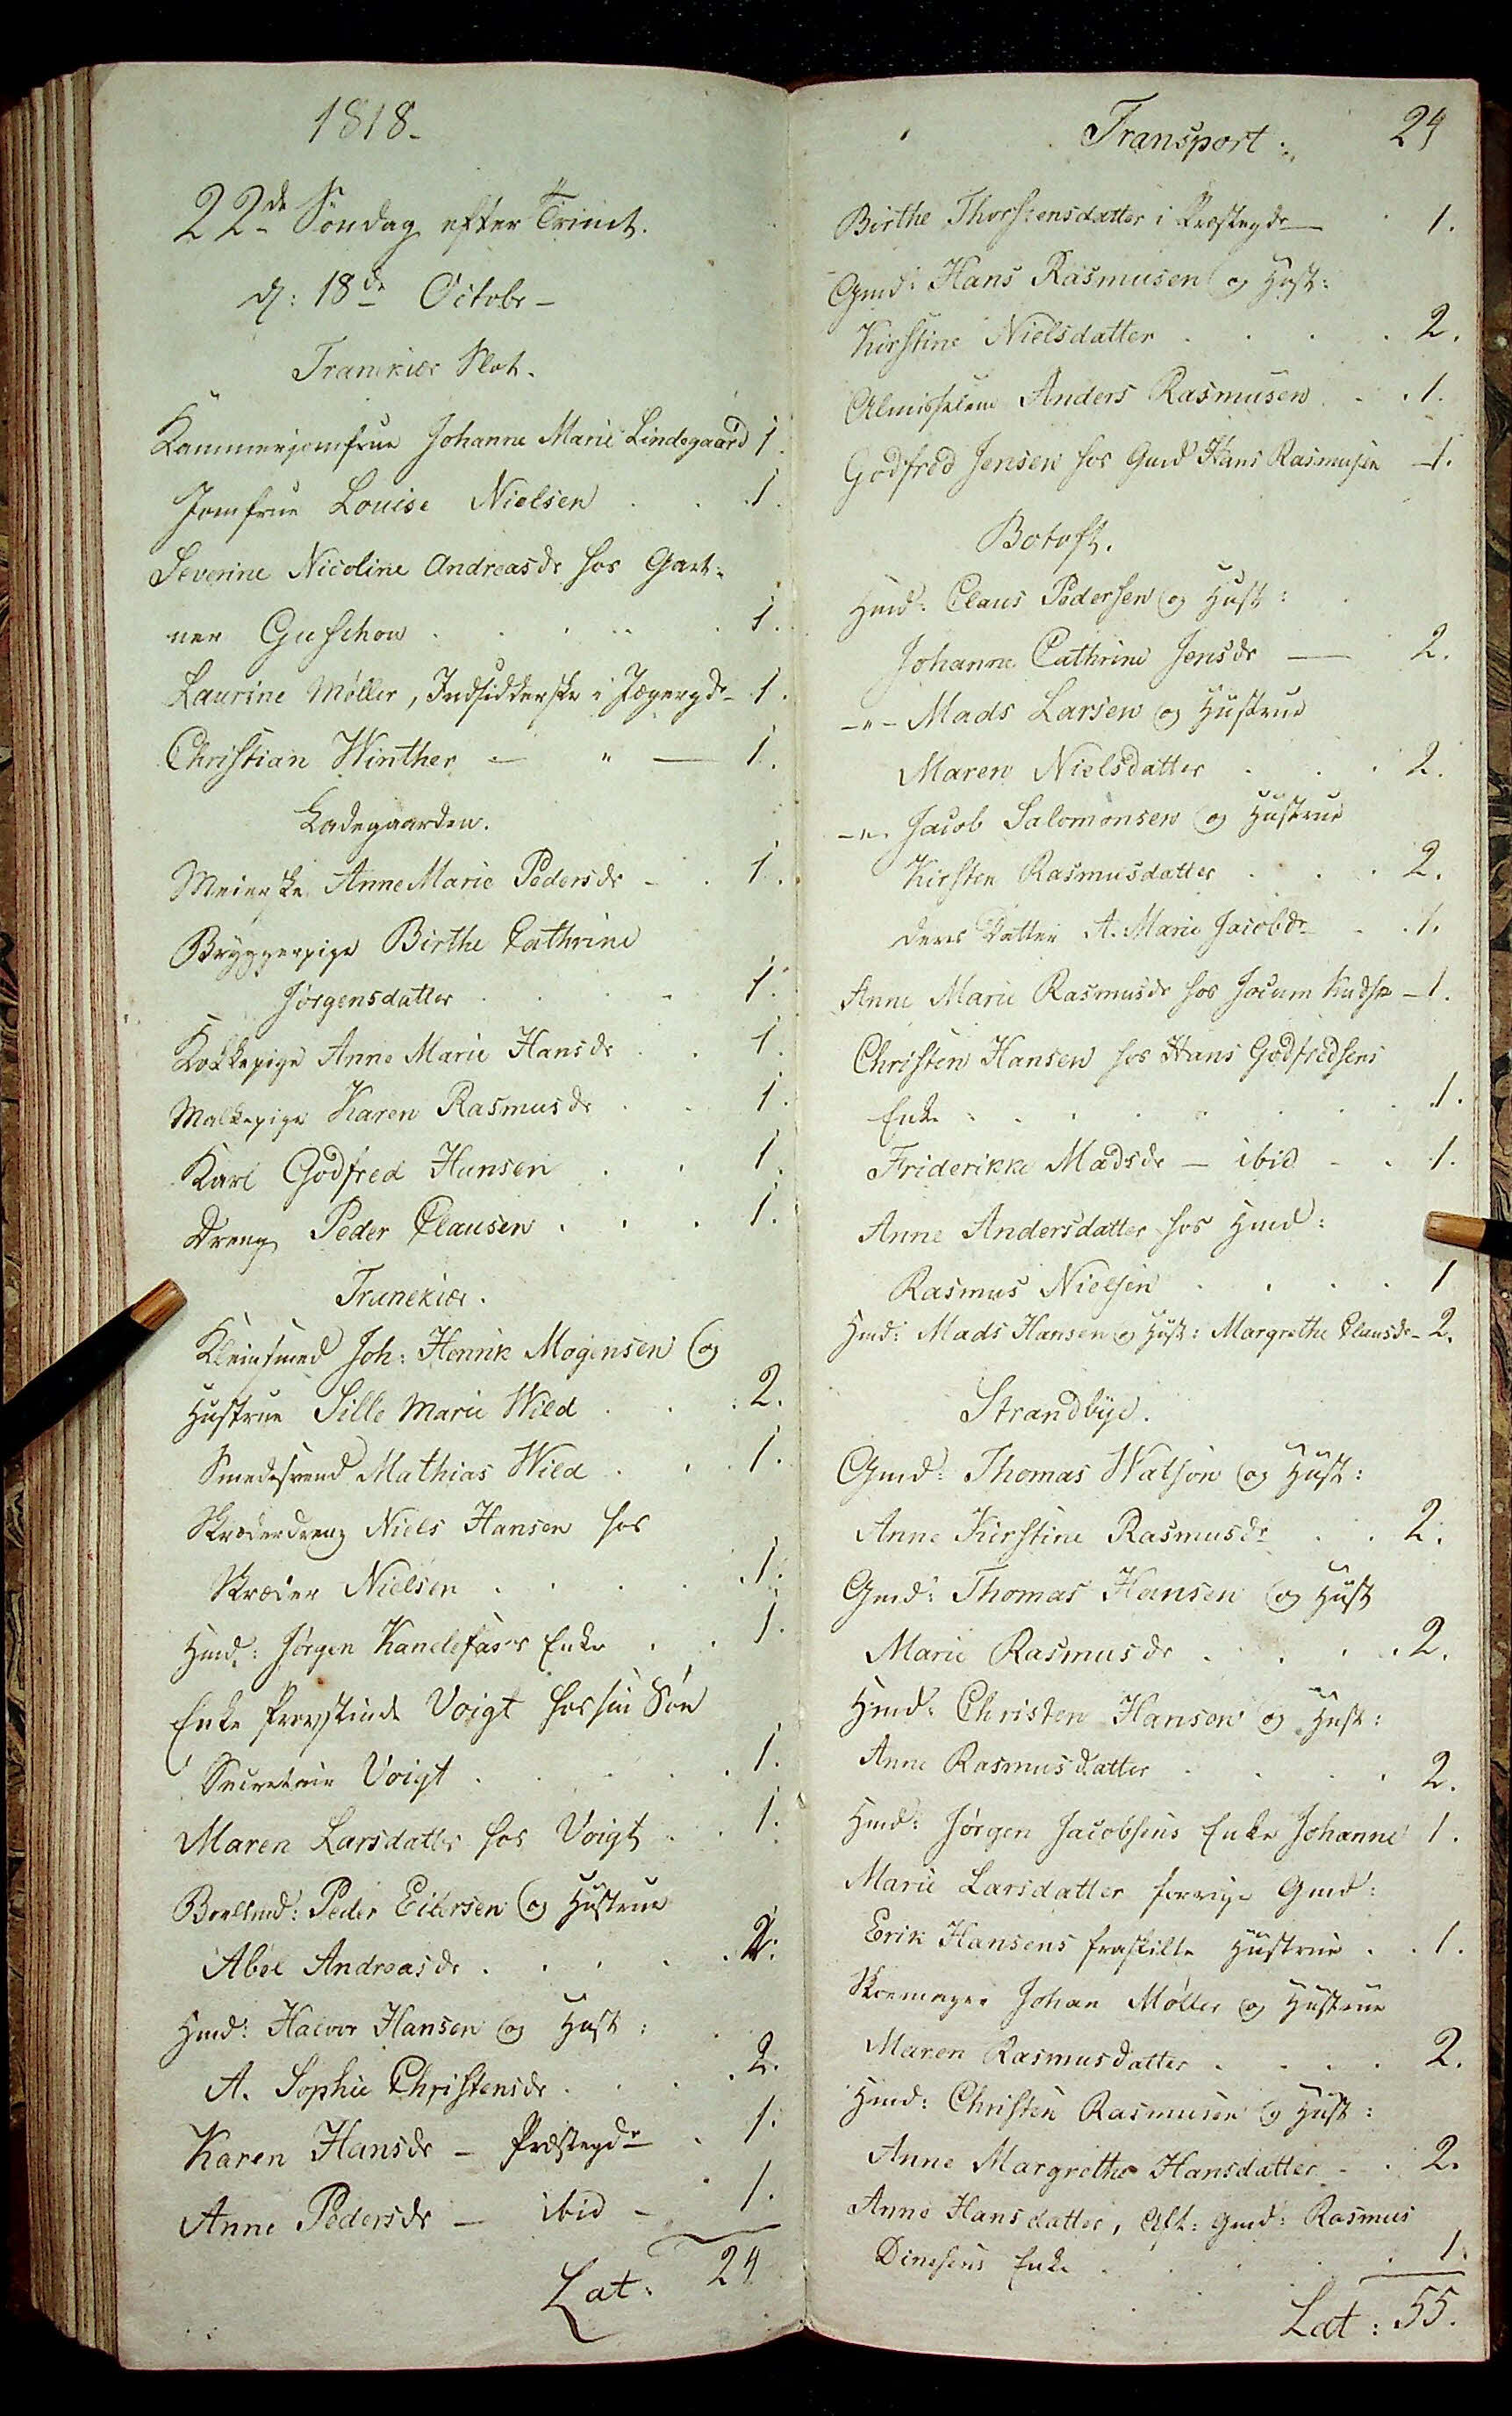1818.
22de Søndag efter Trinit.
d: 18de Octobr-
Tranekiær Slot.
Kammerjomfrue Johanne Marie Lindegaard 1.
Jomfrue Louise Nielsen . . . 1.
Severine Nicoline Andreasdr hos Gart¬
ner Guschou . . . . . . 1.
Laurine Møller, Indsidderse i Jægergdr 1.
Christian Winther -"- 1.
Ladegaarden.
Meierske Anne Marie Pedersdr . . 1.
Bryggerpige Birthe Cathrine
Jørgensdatter . . . . 1.
Kokkepige Anne Marie Hansdr . . 1.
Malkepige Karen Rasmusdr . . 1.
Karl Godfred Hansen . . 1.
Dreng Peder Clausen . . . 1.
Tranekiær.
Kleinsmed Joh: Henrik Mognsen og
Hustrue Sille Marie Wild . . . 2.
Smedesvend Mathias Wild . . 1.
Skræderdreng Niels Hansen hos
Skræder Nielsen . . . . . 1.
Hmd: Jørgen Kanelefas's . .1. Enke
Enke Provstinde Voigt hos sin Søn
Secretair Voigt . . . . . 1.
Maren Larsdatter hos Voigt . . 1.
Boelsmd: Peder Eilersen og Hustrue
Albel Andreasdr . . . . . 2:
Hmd: Hans Hansen og Hust:
A. Sophie Christensdr . . . . 2.
Karen Hansdr - Præstegdr . 1.
Anne Pedersdr - ibid - 1.
Lat: 24
Transport: 24
Birthe Thortensdatter i Præstegdr . 1.
Gmd: Hans Rasmusen og Hust:
Kirstine Nielsdatter . . . . 2.
Almisselem Anders Rasmusen . . . 1.
Godfred Jensen hos Gmd Hans Rasmusen - 1.
Botoft.
Hmd: Claus Pedersen og Hust:
Johanne Cathrine Jensdr - 2.
-"- Mads Larsen og Hustrue
Maren Nielsdatter . . . 2.
-"- Jacob Salomonsen og Hustrue
Kirsten Rasmusdatter . . . 2.
deres Datter A. Marie Jacobdr 1 . . 1.
Anne Marie Rasmusdr hos Jocum Kudsk - 1.
Christen Hansen hos Hans Godfredsens
Enke . . . . . . . . . 1.
Friderikke Madsdr - ibid . . 1.
Anne Andersdatter hos Hmd:
Rasmus Nielsen . . . . 1.
Hmd: Mads Hansen og Hust: Margrethe Clausdr - 2.
Strandbye.
Gmd: Thomas Watson og Hust:
Anne Kirstine Rasmusdr . . 2:
Gmd: Thomas Hansen og Hust
Marie Rasmusdr . . . . 2.
Hmd: Christen Hansen og Hust:
Anne Rasmusdatter . . . . 2.
Hmd: Jørgen Jacobsens Enke Johanne 1.
Marie Larsdatter forrige Gmd:
Erik Hansens fraskilte Hustrue . .1.
Skoemager Johan Møller og Hustrue
Maren Rasmusdatter . . . . 2.
Hmd: Christen Rasmusen og Hust:
Anna Margrethe Hansdatter . . 2.
Anne Hansdatter, Aft: Gmd: Rasmus
Dinesens Enke . . . . . 1.
Lat: 55.
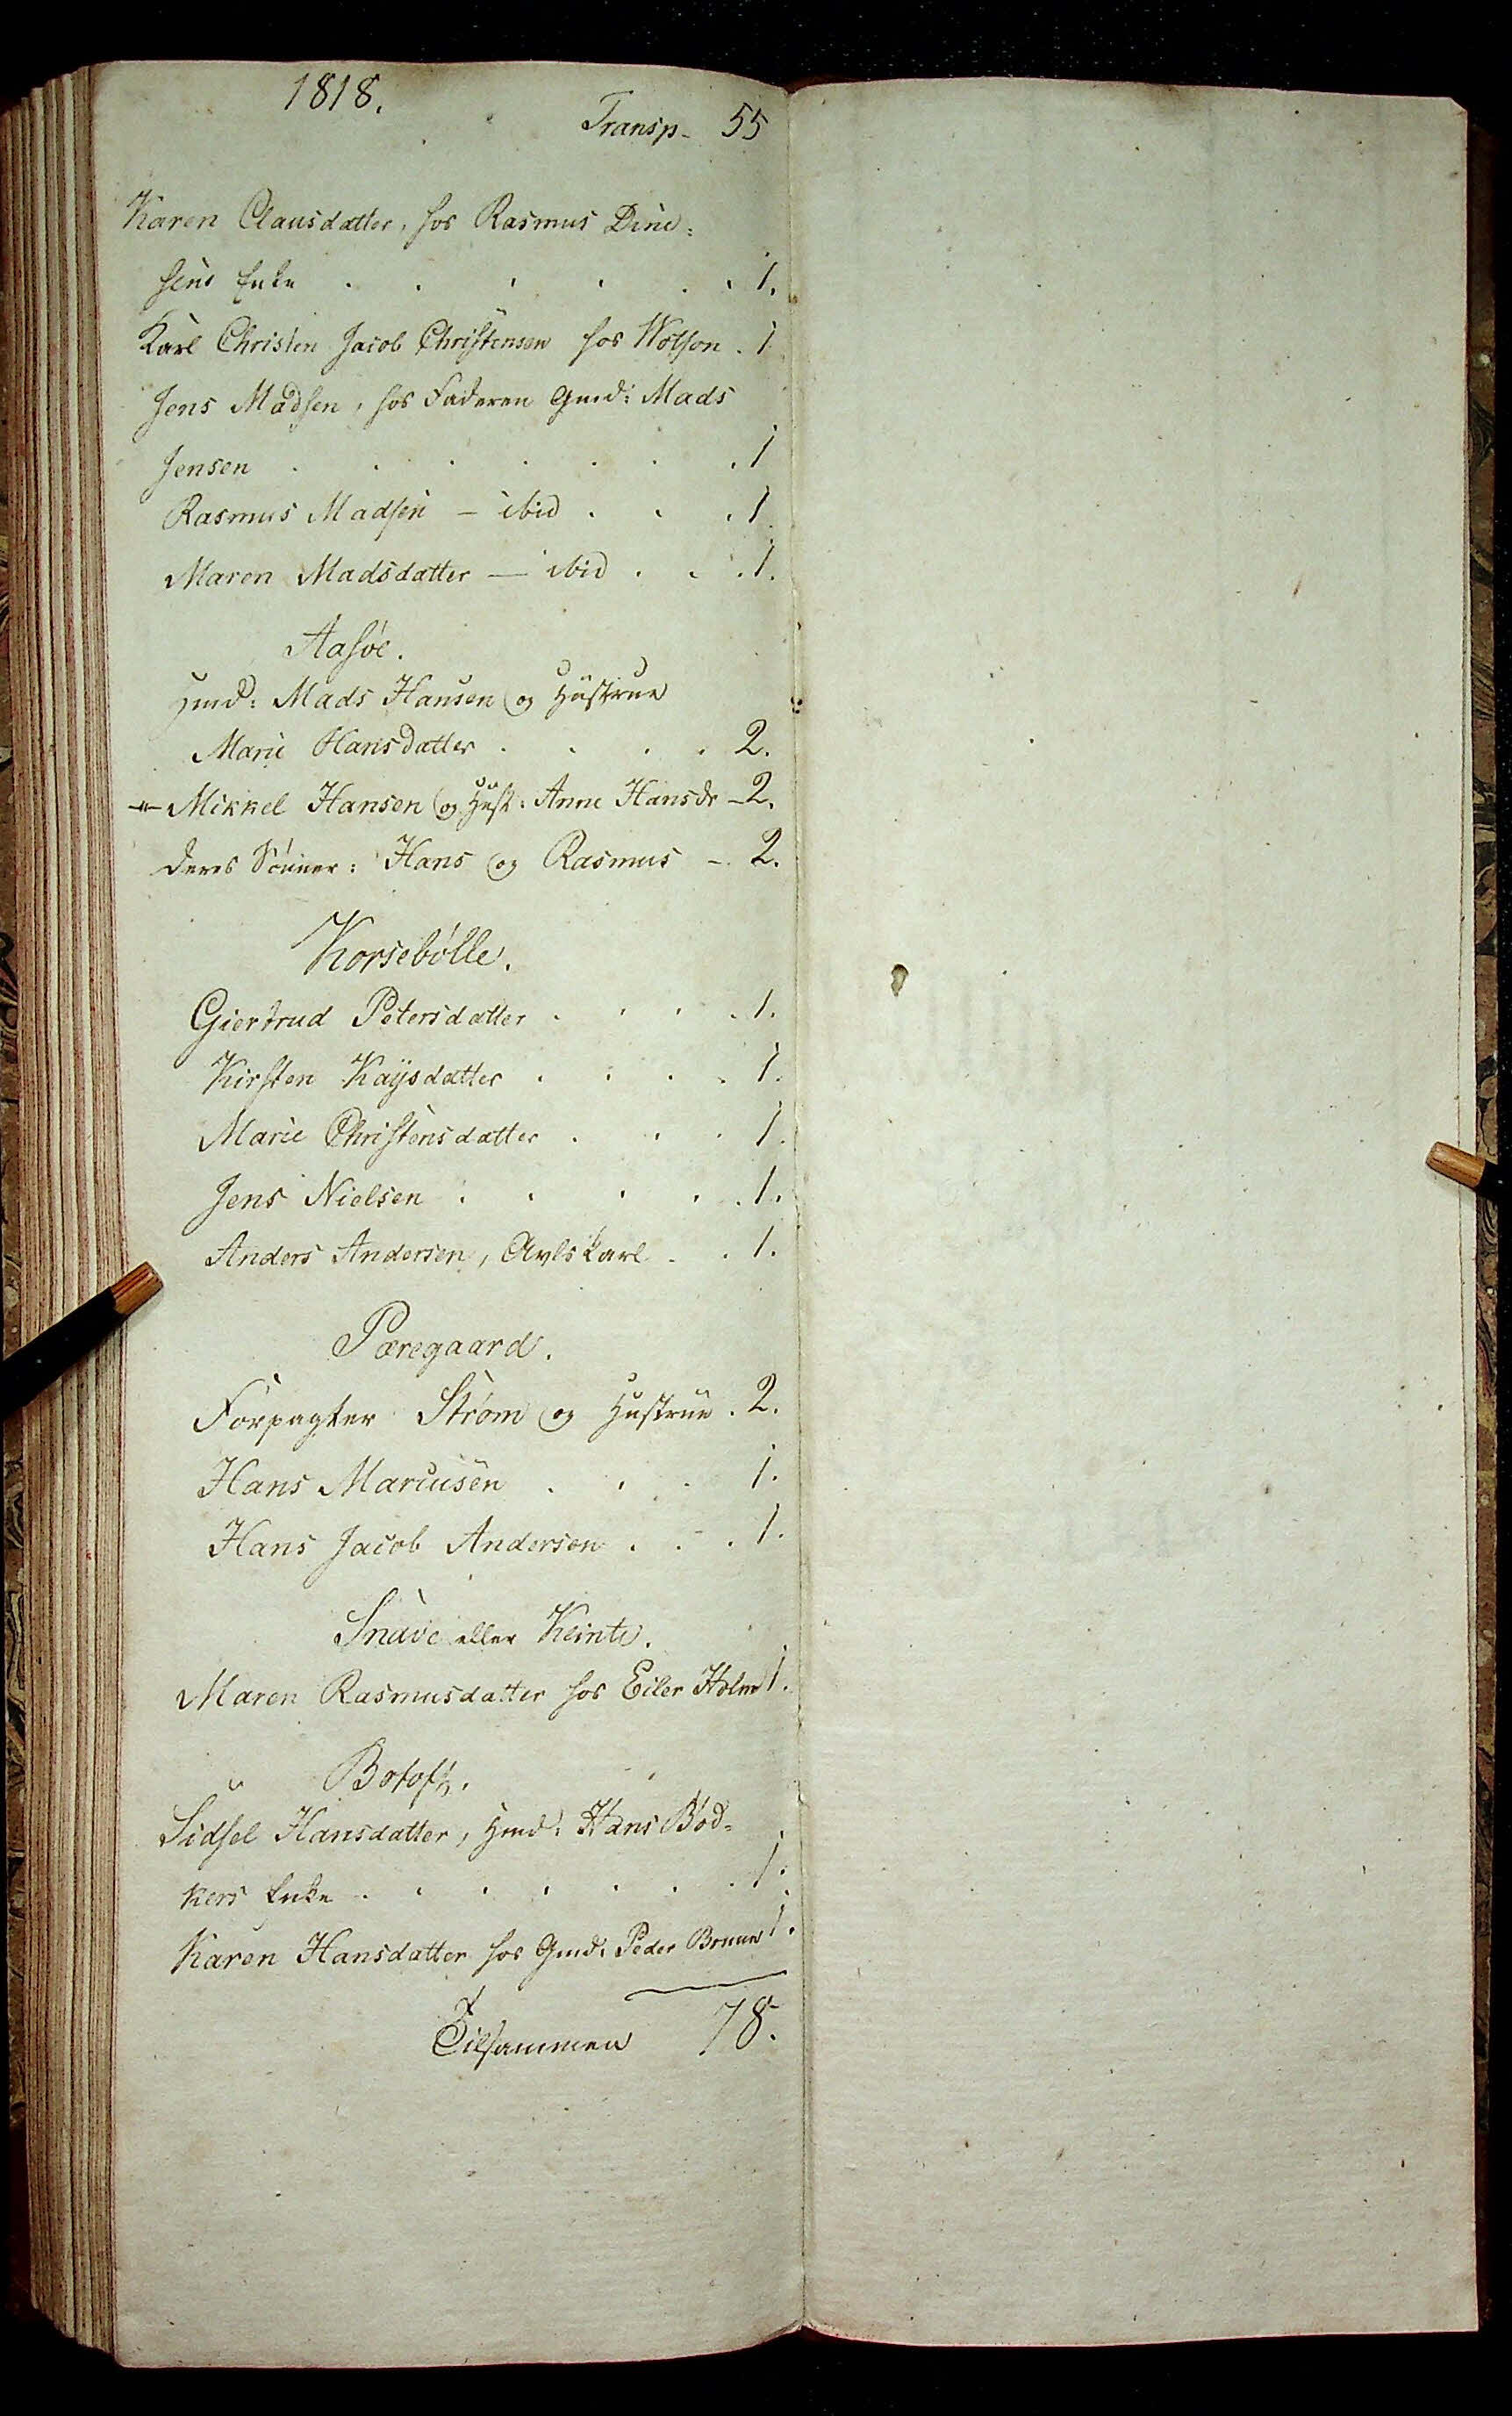1818.
Transp - 55
Karen Clausdatter, hos Rasmus Dine¬
sens Enke . . . . . . 1.
Karl Christen Jacob Christensen hos Watson
Jens Madsen, hos Faderen Gmd: Mads
Jensen . . . . . . . 1
Rasmus Madsen - ibid . . . 1
Maren Madsdatter - ibid . . . 1.
Aasøe.
Hmd: Mads Hansen og Hustrue
Marie Hansdatter . . . . 2.
-"- Mikkel Hansen og Hust: Anne Hansdr - 2.
deres Sønner: Hans og Rasmus - 2.
Korsebølle.
Giertrud Petersdatter . . . . 1.
Kirsten Haysdatter . . . . 1.
Marie Christensdatter . . . 1.
Jens Nielsen . . . . . 1.
Anders Andersen, Avlskarl . . 1.
Pæregaard.
Forpagter Strøm og Hustrue . 2.
Hans Marcusen . . . 1.
Hans Jacob Andersen . . . 1.
Snave eller Klinte.
Maren Rasmusdatter hos Eiler Holm 1.
Botoft.
Sidsel Hansdatter, Hmd: Hans B'd¬
kers Enke . . . . . . . 1.
Karen Hansdatter hos Gmd: Peder Bruun 1.
Tolsammen 78.